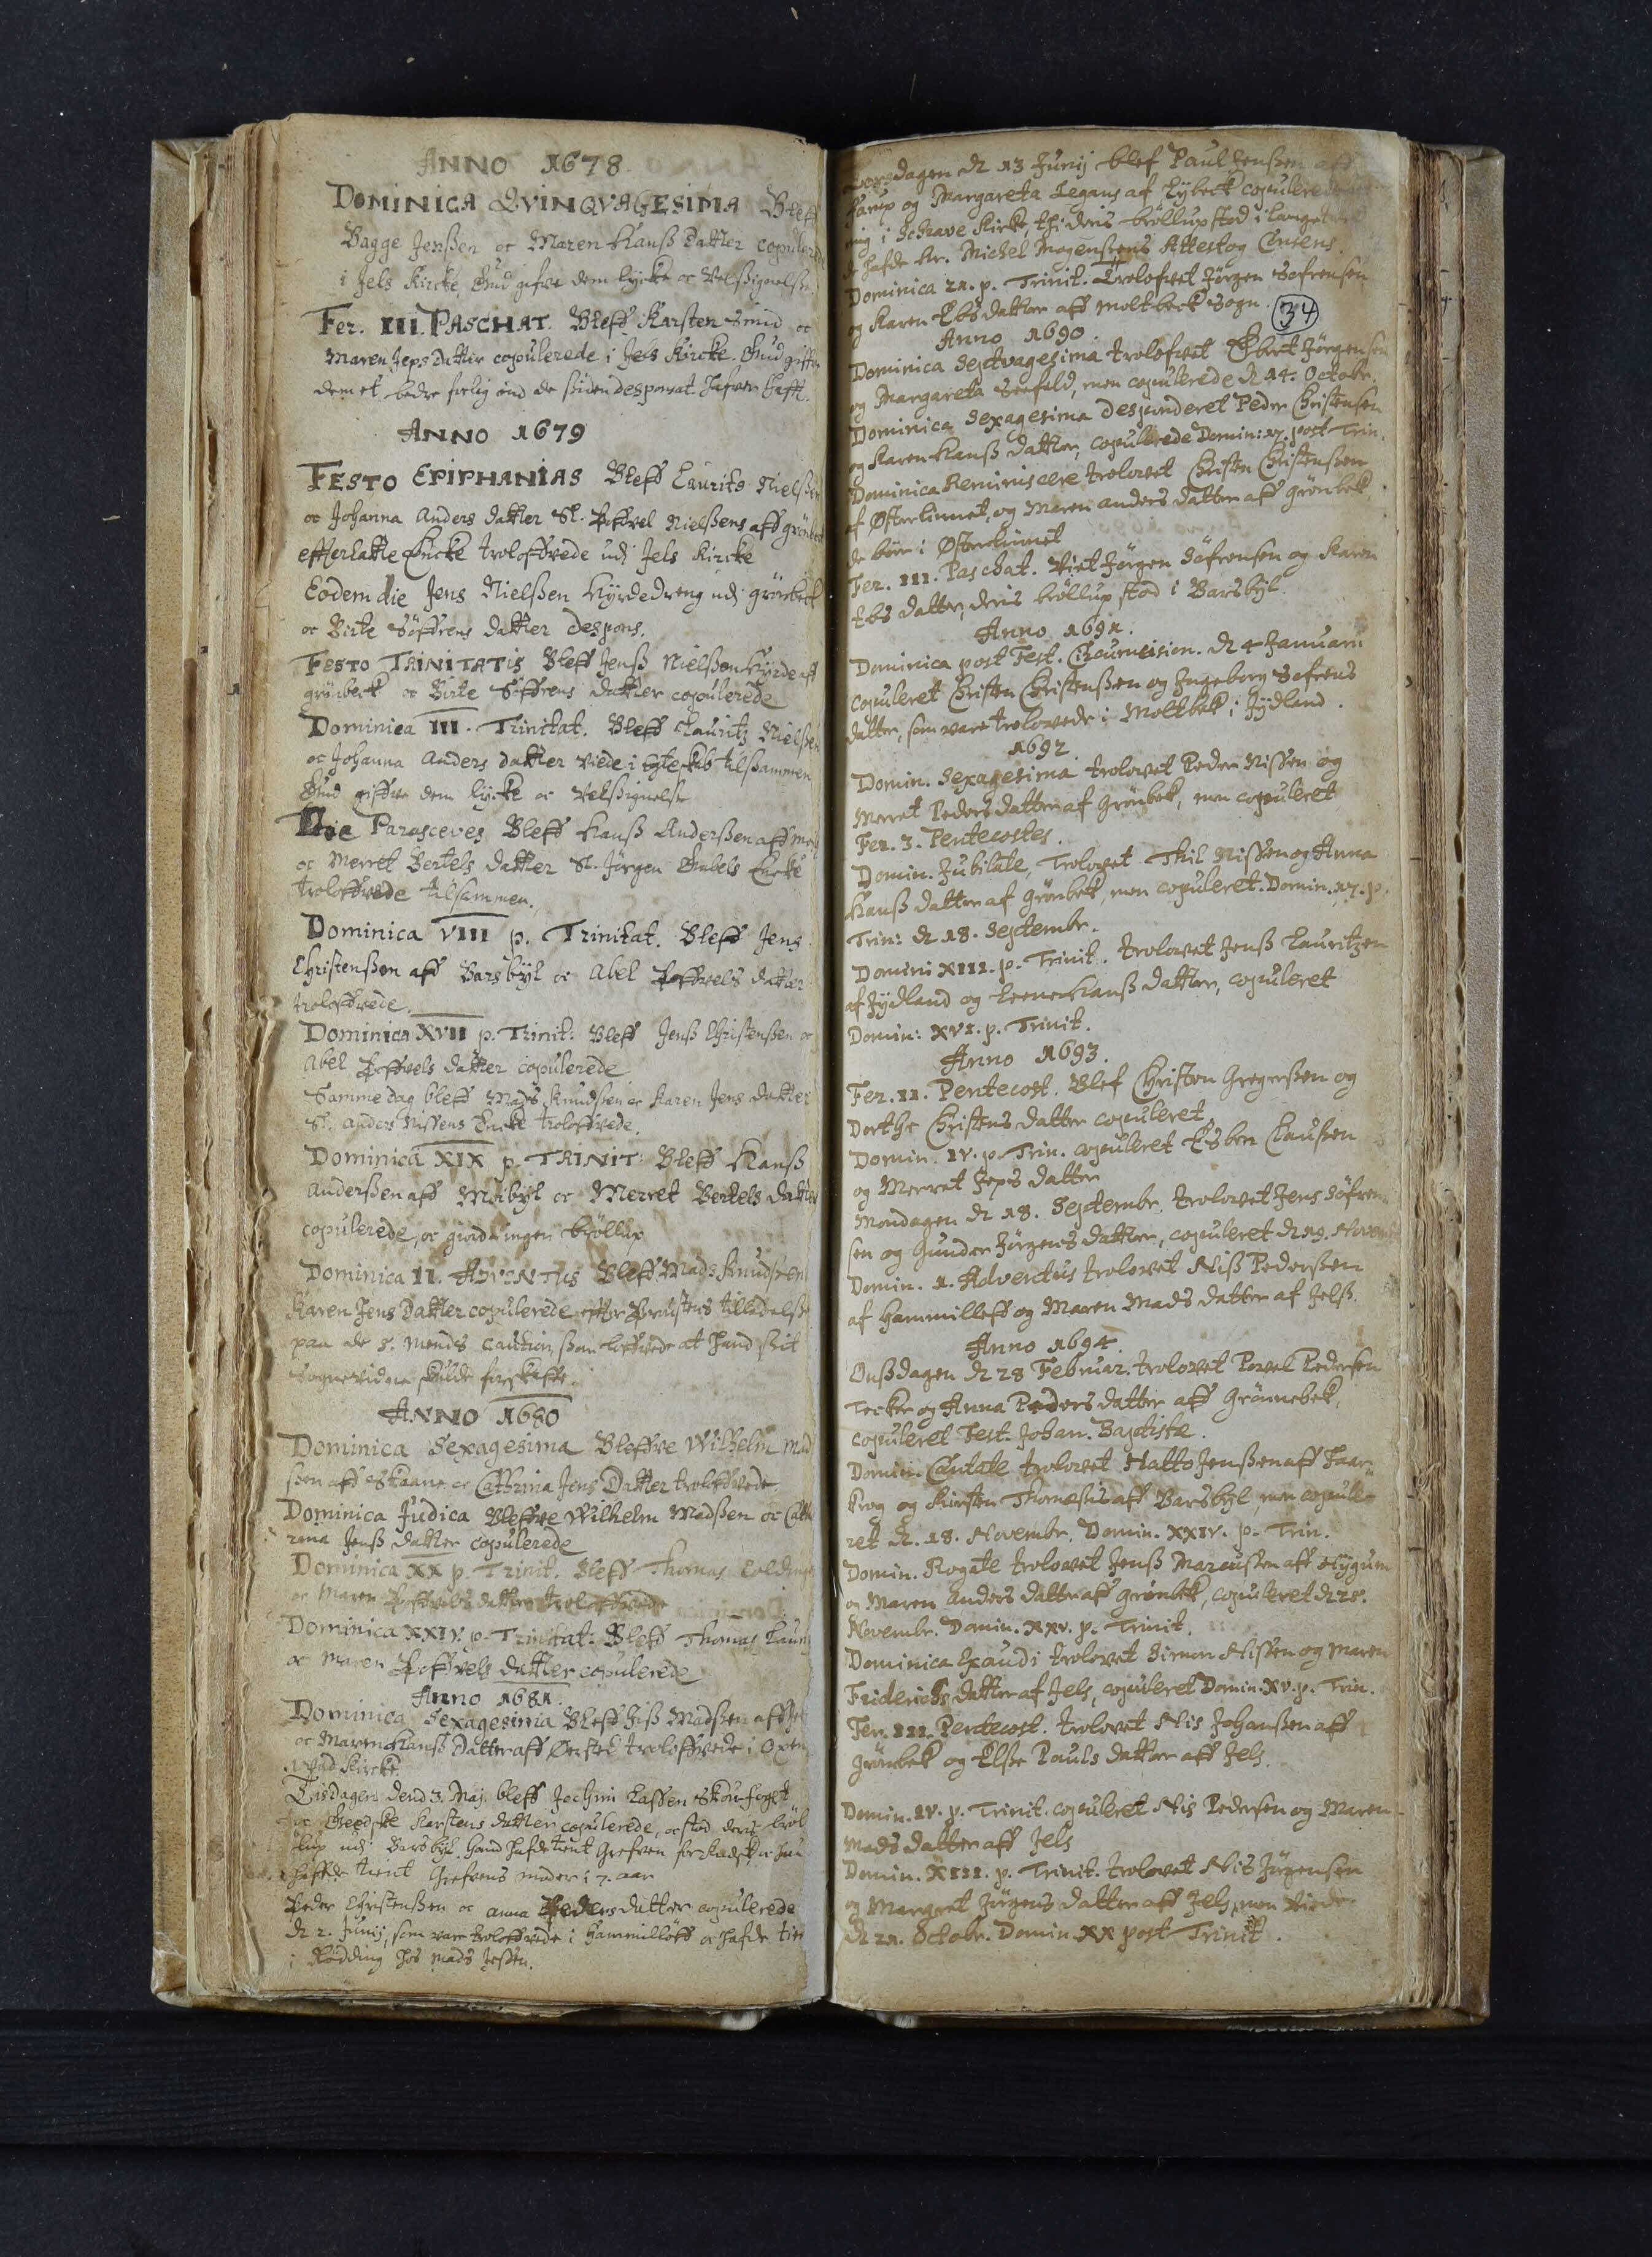ANNO 1678
DOMINICA QVINQVAGESIMA Bleff
Bagge Jenssen oc Maren Hanss Datter copulerede
i Jels Kircke, Gud gifve dem lÿcke oc velsignelse.
Fer. III. PASCHAT. Bleff Karsten Smid oc
Maren Jeps Datter copulerede i Jels Kircke. Gud giffve
dem et bedre forlig ind de ssiden desponat hafver hafft.
ANNO 1679
FESTO EPIPHANIAS Bleff Laurits Nielssen
oc Johanna Anders Datter Sl. Pofvel Nielssens aff Grønbeck
effterladtte Encke troloffvede udi Jels Kircke
Eodem die Jens Nielssen Hyrdedreng udi Grønbeck
oc Birte Søffrens Datter despons.
FESTO TRINITATIS Bleff Jenss Nielssen Hyrde aff
Grønbeck oc Birte Søffrens Datter copulerede
Dominica III Trinitat. Bleff Lauritz Nielssen
oc Johanna Anders Datter viede I Egteskab tilsammen
Gud giffve dem Lycke oc Velsignelse
Die Parasceves Bleff Hanss Anderssen aff
oc Merret Bertels Datter Sl. Jørgen Gabels Encke
troloffvuede tilsammen.
Dominica VIII p. Trinitat. Bleff Jens
Christenssen aff Barsbyl oc Abel Poffvels Datter
troloffvede.
Dominica XVII p. Trinit. Bleff Jenss Christenssen oc
Abel Poffvels Datter copulerede
Samme Dag bleff Mads Knudssen oc Karen Jens Datter
S. Anders Nissens Encke trolofvede.
Dominica XIX p. TRINIT: Bleff Hanss
Anderssen aff Moibÿl oc Merret Bertels Datter
copulerede oc giord ingen brøllup
Dominica II. ADVENTUS Bleff Mads Knudsen
Karen Jens Datter copulerede effter Prustens tilladelse
paa de## s. mands caution ssom loffvede## at Hand ssit
Sognevid## skulde f##skaffe
ANNO 1680
Dominica Sexagesima Bleffve Wilhelm Mad
sen aff Skaane oc Cathrina Jens Datter troloffvede.
Dominica Judica Bleffve Wilhelm Madsen oc Cath
rina Jenss Datter copulerede
Dominica XX p. Trinit. Bleff Thomas Colding
oc Maren Poffvels Datter troloffvede
Dominica XXIV. p. Trinit: Bleff Thomas Lau
oc Maren Poffvels Datter copulerede
Anno 1681.
Dominica Sexagesima Bleff Jess Madssen aff
oc Maren Hanss Datter aff Ørsted troloffvede i Oxen¬
vad Kircke.
Tisdagen dend 3. Maj bleff Jochim Lassen Skoufoget
oc Geedske Karstens Datter copulerede, oc stod deres brøl
lup udi Barsbÿl. Hand hafde tient Grefven for kudsk oc hun
haffde tient Grefvens moder i 7. aar
Peder Christenssen oc Anna Pedersdatter copulerede
d 2. Junij, som var troloffvede i Hammilløff oc hafde tie 
i Rødding hos Mads Jessen.
34
Torsdagen d 13 Junij blef Paul Jensen aff
Farup og Margareta Legans aff Lybeck copulere
i Schrave Kircke, thi deris brøllup stod i Lange
de hafde hrh Mickel Mogenssens
Dominica 21. p. Trinit. Trolofvet Jørgen Sofrensen
og Karen Ebsdatter aff Moltbeck Sogn.
Anno 1690.
Dominica Septuagesima trolofvet Ebert Jørgensen
og Margareta Seefeld, men copulerede d 14. Octobr.
Dominica Sexagesima despunderet Peder Christensen
og Karen Hanss Datter, copulerede Demin: 17. post Trin.
Dominica Reminiscere trolovet Christen Christenssen
af Østerlinnet og Maren Anders Datter aff Grønbek
de boer i Østerlinnet
Fer. III. Paschat. Viet Jørgen Søfrensen og Karen
Ebs datter, deris brøllup stod i Barsbyl.
Anno 1691.
Dominica post Fest. Circumcision. d 4 Januari
copuleret Christen Christenssen og Ingeborg Sofrens
Datter, som vare trolovede i Moltbek i Jydland.
1692.
Domin. Sexagesima trolovet Peder Nissen og 
Merret Peders Datter aff Grønbek, men copuleret
Fer. 3. Pentecostes
Domin. Jubilate Trolovet Thil Nissen og Anna
Hanss Datter af Grønbek, men copuleret. Domin. 17. p.
Trin: d 18. Septembr.
Domini XIII. p. Trinit. trolovet Jenss Lauritzen
af Jydland og Leene Hanss Datter, copuleret
Domin: XVI. p. Trinit.
Anno 1693.
Fer. II. Pentecost. Blef Christen Gregerssen og
Dorthe Christens Datter copuleret.
Domin. IV. p. Trin. copuleret Esben Clausen
og Merret Jeps Datter
Mandagen d 18. Septembr. Trolovet Jens Søfren¬
sen og Gunder Jørgens Datter, copuleret d 19.
Domin. 1. Adventus trolovet Niss Pederssen
af Hammilleff pg Maren Mads Datter aff Jels.
Anno 1694.
Onssdagen d 28 Februar trolovet Povel Pedersen
Tecker og Anna Peders Datter aff Grønnebek,
copuleret Fest. Johan. Baptiste.
Domin. Cantate trolovet Hatto Jenssen af Faar¬
krog og Kirsten Thomæssis aff Barsbyl, men copule¬
ret d. 18. Novembr. Domin. XXIV. P. Trin.
Domin. Rogate trolovet Jenss Marcussen aff Hygum
og Maren Anders Datter aff Grønbek, copuleret d 25.
Novembr. Domin. XXV. p. Trinit.
Dominica Exaudi trolovet Simen Nissen og Maren
Friderichs Datter af Jels, copuleret Domin. XV. p. Trin.
Fer. III. Pentecost. trolovet Nis Johanssen aff
Grønbek og Elsse Pauls Datter aff Jels.
Domin. IV. p. Trinit. copuleret Nis Pedersen og Maren
Mads Datter aff Jels.
Domin. XIII. p. Trinit trolovet Nis Jørgensen
og Margret Jørgens Datter aff Jels, men viede
d 21. Octobr. Domin. XX post Trinit.
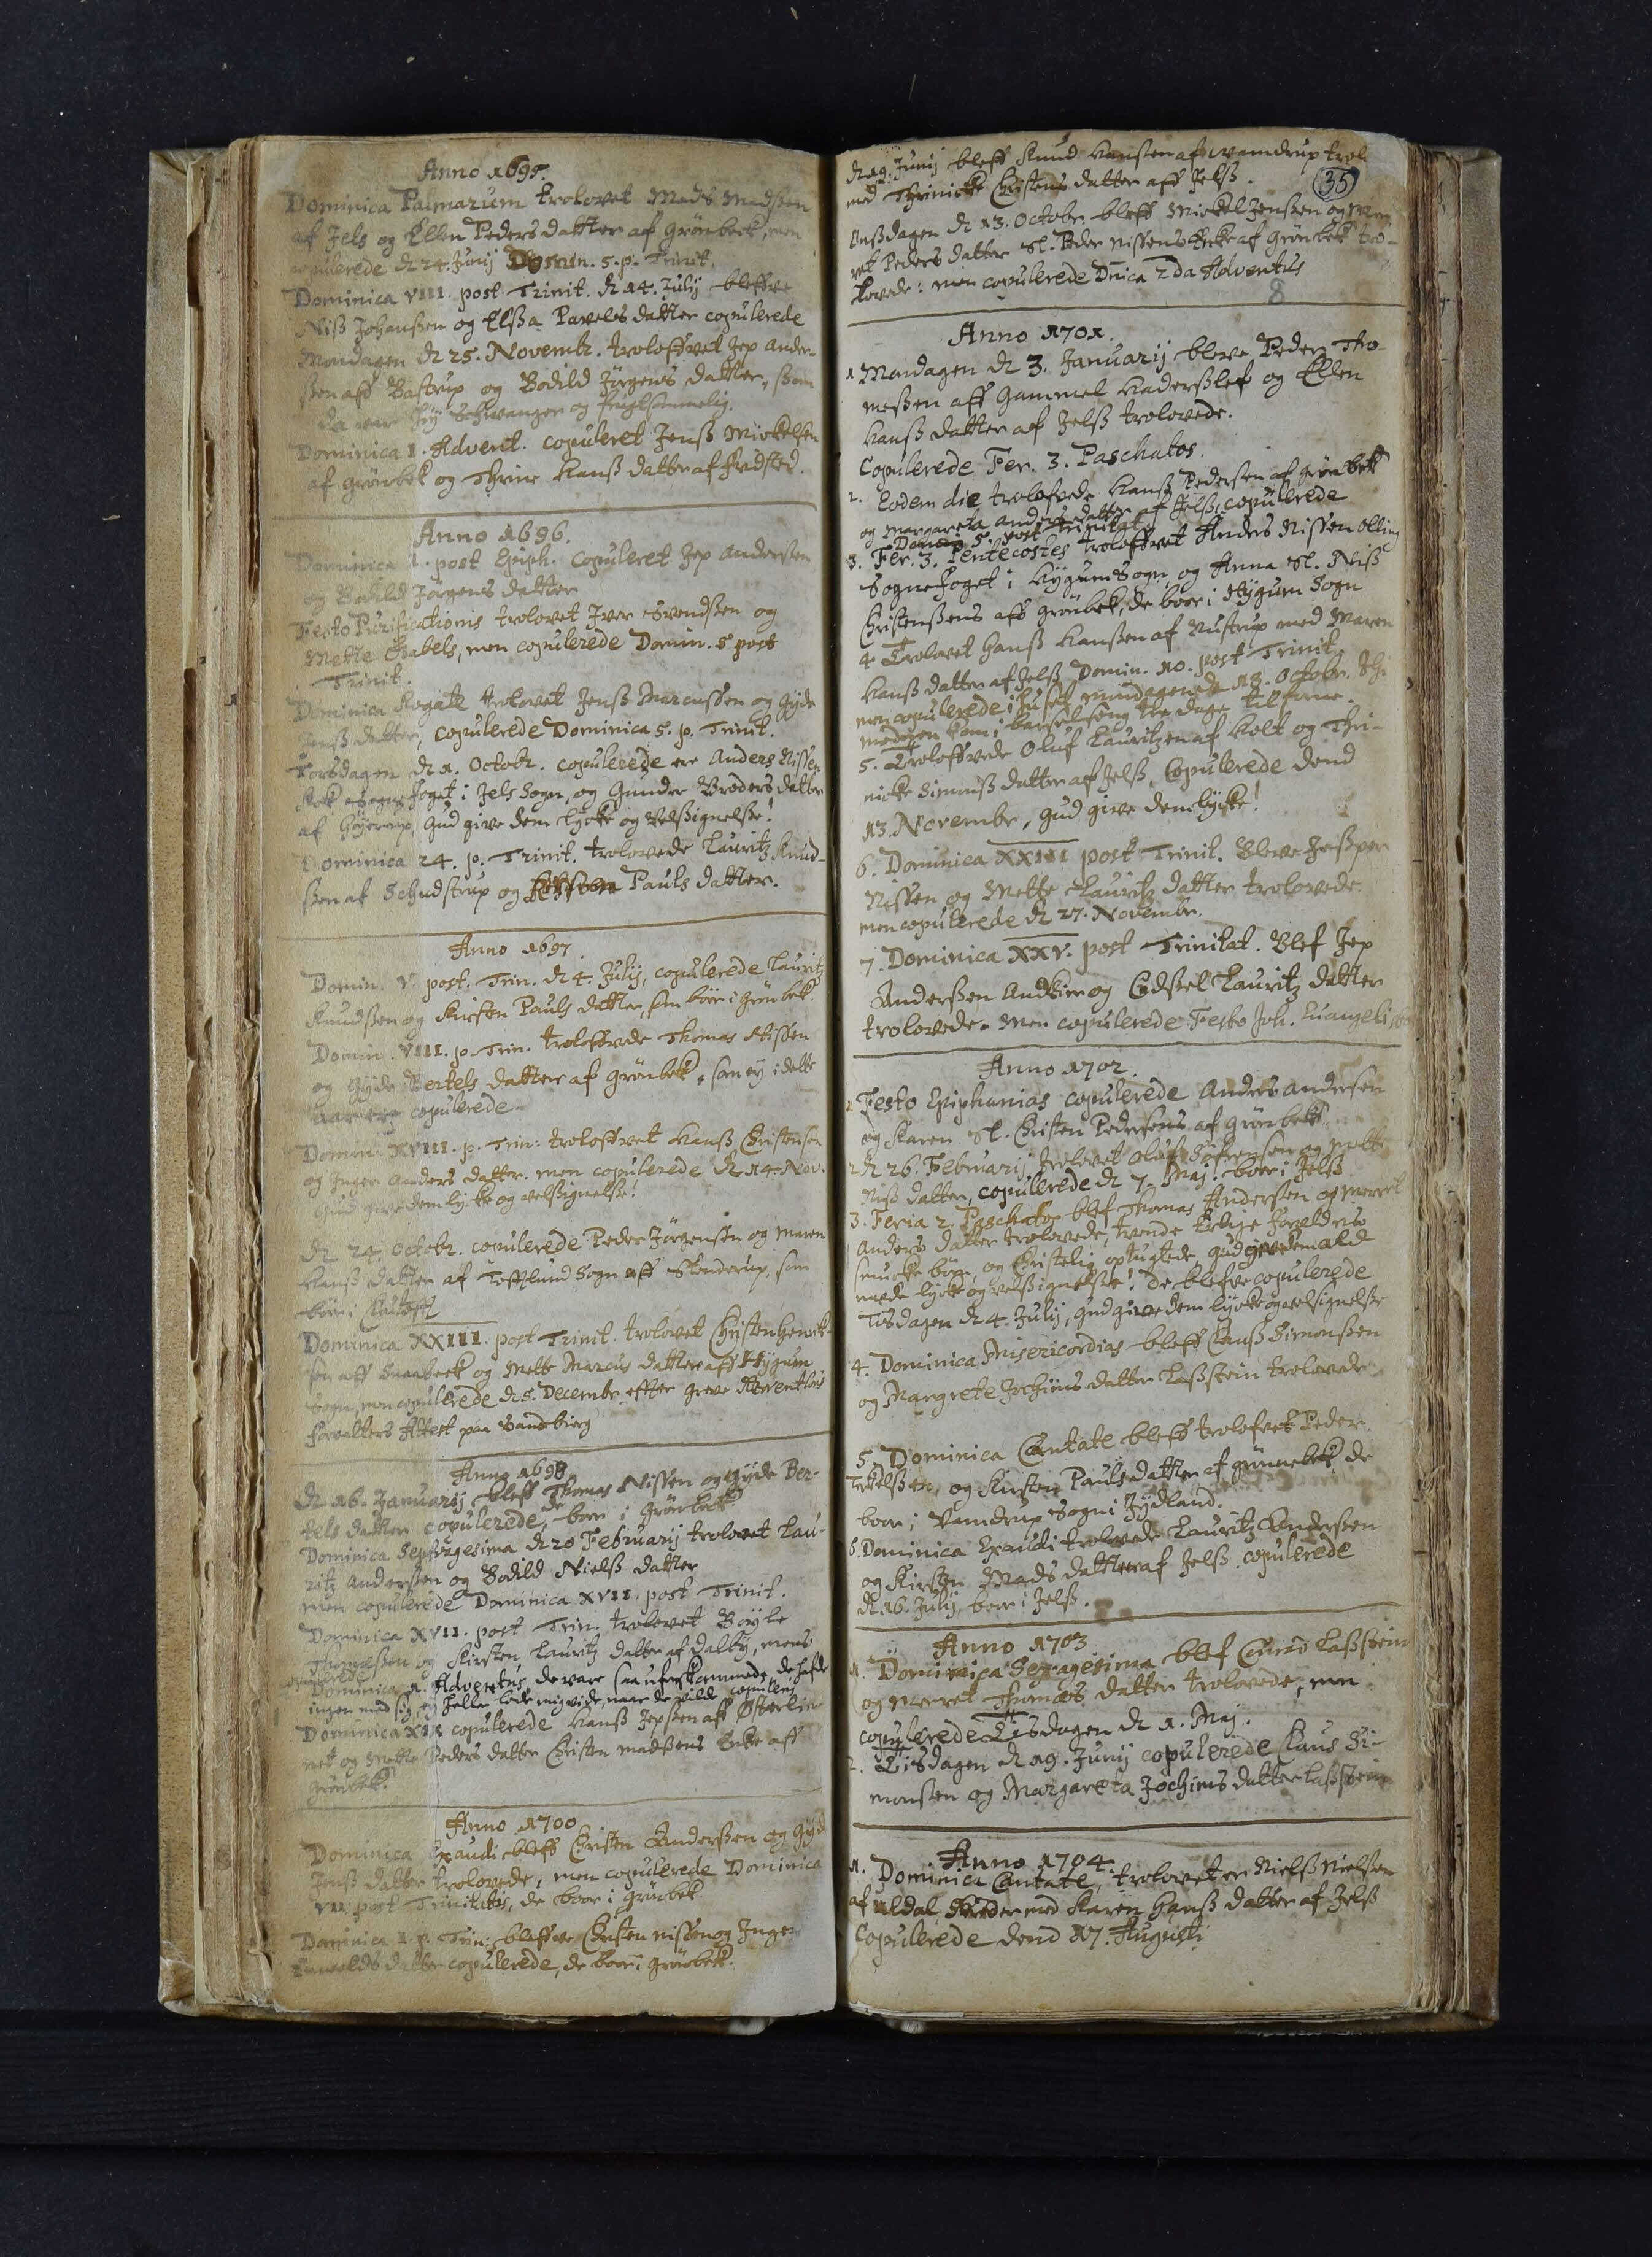Anno 1695.
Dominica Palmarum trolovet Mads Madsen 
af Jels og Ellen Peders Datter aff Grønbeck, men
copulerede d 24. Junij Domin. 5. p. Trinit.
Dominica VIII post Trinit. d 14 Julij bleffve
Niss Johanssen og Elssa Povels Datter copulerede.
Mandagen d 25. Novembr. trolofvet Jep Ander¬
ssen aff Bastrup og Bodild Jørgens Datter, ssom
da var høy Schvanger og Frugtsommelig.
Dominica 1. Advent. Copuleret Jenss Mickelsen
aff Grønbeck og Thrine Hans Datter af Fridsted.
Anno 1696.
Dominica 1. post Epiph. copuleret Jep Andersen
og Bodild Jørgens Datter
Festo Purificationis trolovet Iver Svendssen og
Mette Gabels, men copulerede Domin. 5 post 
Trinit.
Dominica Rogate trolovet Jenss Marcussen og Gyde
Jens Datter, copulerede Dominica 5. p. Trinit.
Torsdagen d 1. Octobr. copulerede ere Anders Nissen
Kok Sognefoget i Jels Sogn, og Gunder Broders Datter
af Høyerup, Gud give dem lycke og velsignelse!
Dominica 24. p. Trinit. trolovede Lauritz Knud¬
ssen af Schudstrup og Kirsten Pauls Datter.
Anno 1697
Domin. V. post Trin. d 4. Julij, copluerede Lauritz
Knudssen og Kirsten Pauls Datter, som boer i Grønbek.
Domin. VIII. P. Trin. trolofvede Thomas Nissen
og Gyde Bertels Datter af Grønbek, som ey i dette
aar ere copulerede.
Domin. XVIII. p. Trin: trolofvet Hanss Christensen
og Inger Anders Datter, men copulerede d 14. Nov.
Gud give dem lycke og velsignelse!
D 24. Octobr. copulerede Peder Jørgensen og Maren
Hanss Datter af Tofftlund Sogn aff Stenderup, som
boer i Clatofft
Dominica XXIII post Trinit. trolovet Christen Henrick¬
sen aff Scar##beck og Mette Marcus Datter aff Hygum
Sogn, men copulerede d 5. Decembr effter Greve Rewentlovs
Forvalters Attest paa Sandbierg
Anno 1698
D 16. Januarij bleff Thomas Nissen og Gyde Ber¬
tels Datter copulerede, boer i Grønbeck.
Dominica Septuagesima d 20 Februarij trolovet Lau¬
ritz Anderssen og Bodild Niels Datter
men copulerede Dominica XVII. post Trinit.
Dominica XVII. post Trin. trolovet Boyle
Thomæssen og Kirsten Lauritz Datter af Dalby, mens
copulerede
Dominica 1. Adventus de var saa uforskammede, de hafde
ingen med sig, ey heller lode mig vide, naar de vilde copuleris
Dominica XIX copulerede Hanss Jepssen aff Østerlin¬
net og Mette Peders Datter Christen Madsens Enke aff
Grønbeck.
Anno 1700
Dominica Exaudi bleff Christen Andersen og Gyde
Jens Datter trolovede, men copulerede Dominica
VII post Trinitatis, de boer i Grønbek
Dominica 1 p. Trin: bleffve Christen nissen og Inger
Envolds Datter copulerede, de boer i Grønbek.
35
d 19. Junij bleff Knud Hanssen af vamdrup trol 
med Thrinicke Christens Datter aff Jelss.
Onsdagen d 13. oktober bleff Mickel Jenssen og Mer¬
ret Peders Datter Sl. Peder Nissens Encke aff Grønbek tro¬
lovede: men copulerede Dnica 2da Adventus
Anno 1701.
1 Mandagen d 3. Januarij blev Peder Tho¬
massen aff Gammel Hadersslef og Ellen
Hanss Datter af Jelss trolovede.
Copulerede Fer. 3. Paschatos.
2. Eodem die trolofede Hanss Pedersen af Grønbek
og Margaretha Anders Datter af Jelss, copulerede
Domin 5. post Trinitat.
3. Fer. 3. Pentecostes troloffvet Anders Nissen Olling
Sognefoget i Hÿgum Sogn og Anna Sl. Niss
Christensens aff Grønbek, de boer i Hygum Sogn
4. Trolovet Hanss Hanssen af Nustrup med Maren
Hanss Datter af Jelss Domin. 10. post Trinit.
men copulerede i huset mandagen d 18. Octobr. Thi
moderen kom i barselseng tre dage tilforne.
5. Troloffede Oluf Lauritzen af Holt og Thri¬
nicke Simenss Datter af Jelss, Copulerede dend
13 Novembr, Gud give dem lycke!
6. Dominics XXIII post Trin. Bleve Jessper
Nissen og Mette Lauritz Datter trolovede
men copulerede d 27. Novembr.
7. Dominica XXV. post Trinitat. Blef Jep
Andersen Andkier og Cidssel Lauritz Datter
trolovede. men copulerede Festo Joh. Euangeli
Anno 1702
1. Festo Epiphanias copulerede Anders Andersen
og Karen Sl. Christen Pedersens af Grønbek
2. d 26 Februarij trolovet Oluf Søfrensen og Mette
Niss Datter, copulerede d 7. Maj. boer i Jelss
3. Feria 2. Paschatos blef Thomas Andersen og Merret
Anders Datter trolovede, tvende erlige foreldris
smucke børn, og christelig optugtede Gud 
naade lycke og velsignelse! De blefve copulerede
Tisdagen d 4. Julij, Gud give dem lycke og velsignelse.
4. Dominica Misericordias bleff Clauss Simonssen
og Margrete Jochims Datter Lasstein trolovede
5. Dominica Cantate bleff trolovet Peder
Terckelssen, og Kirsten Pauls Datter af grønnebek de
boer i Vamdrup Sogn i Jydland.
6. Dominica Exaudi trolovede Lauritz Anderssen
og Kirsten Mads Datter af Jelss. copulerede
d 16. Julij. boer i Jels.
Anno 1703.
1. Dominica Sexagesima blef Conrad Lasstein
og Merret Thomæs datter trolovede, men
copulerede Tisdagen d 1. Maj.
2. Tisdagen d 19. Junij copulerede Claus Si¬
monssen og Margareta Jochims Datter Lasstein.
Anno 1704.
1. Dominica Cantate trolovet er Nielss Nielsen
af Uldal Skreder med Karen Hanss Datter af Jelss
copulerede dend 17. Augusti